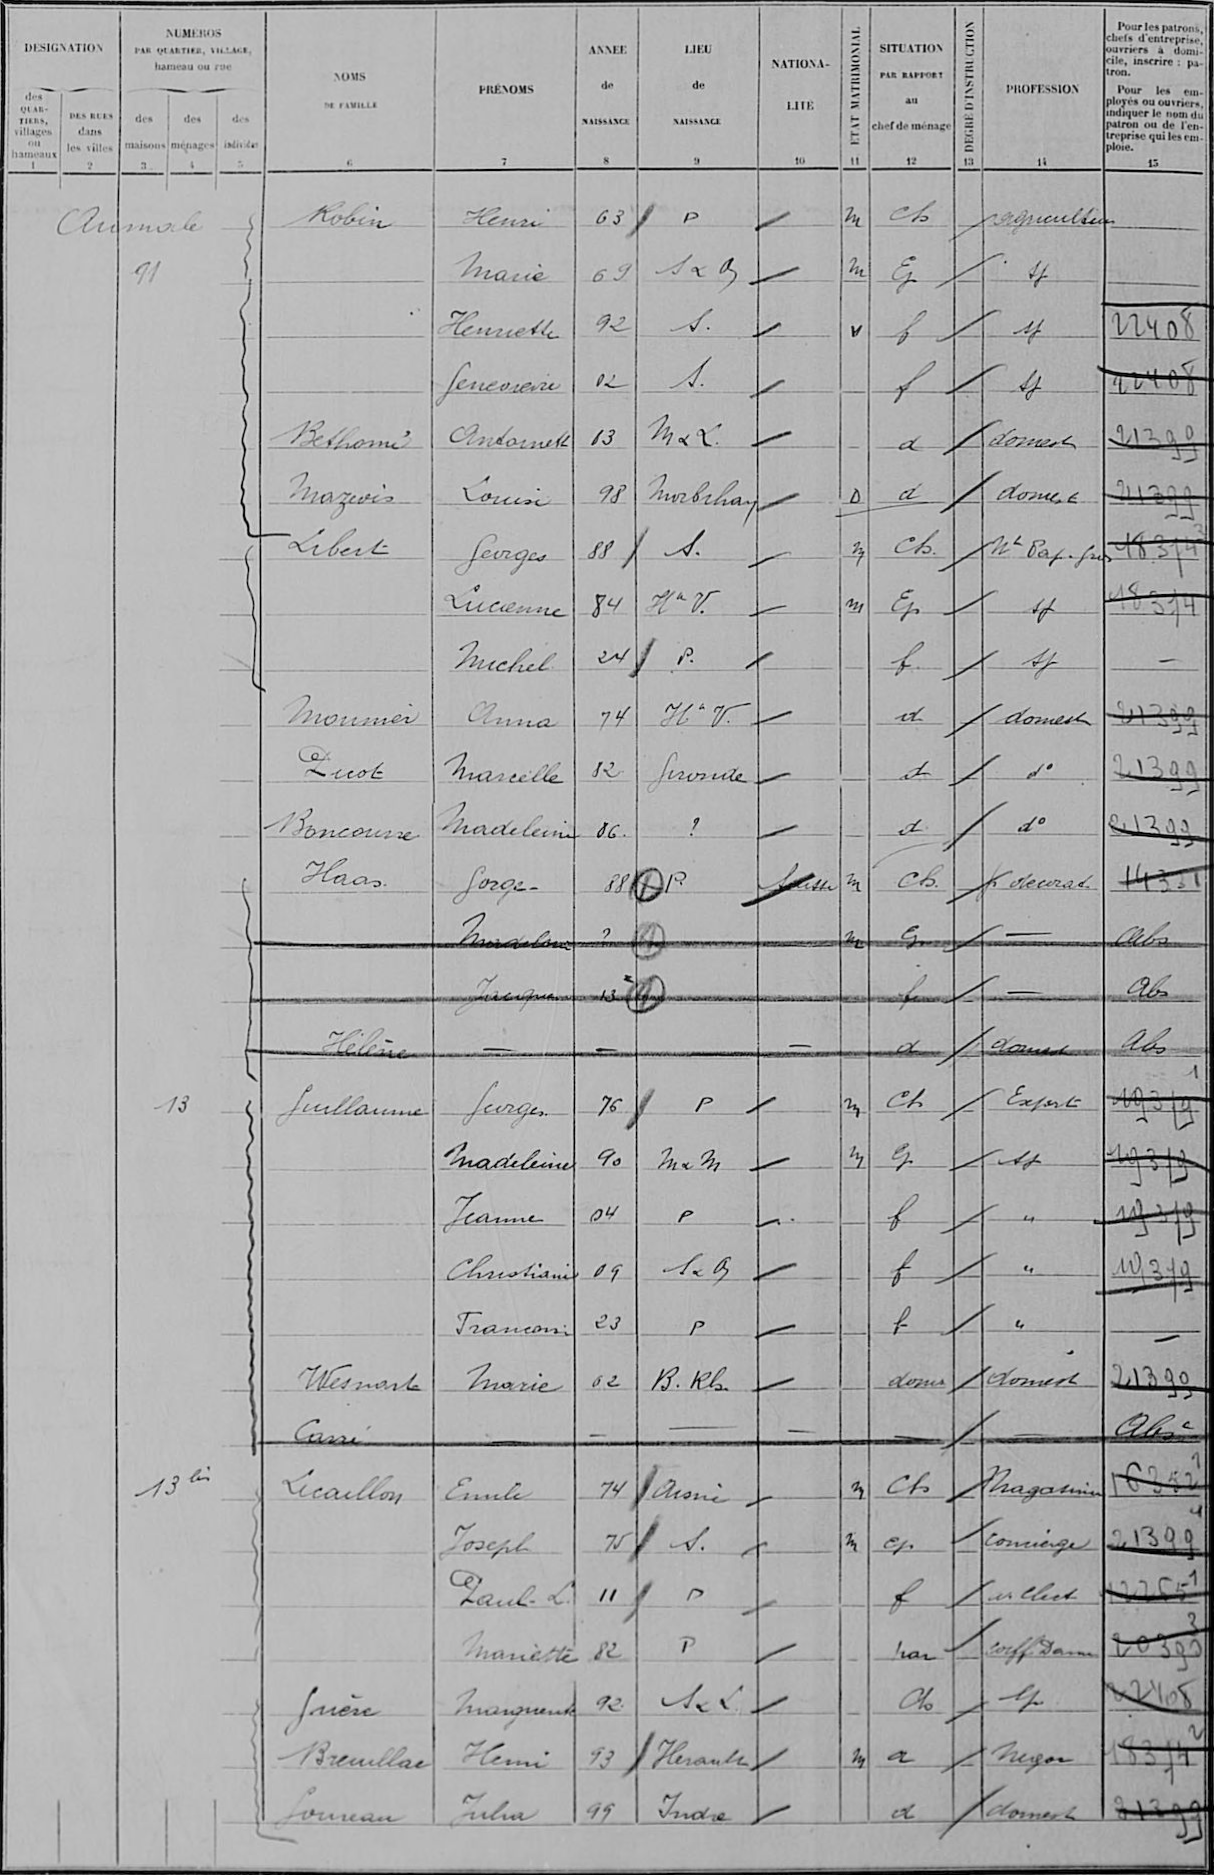Robin/Henri/63/P/¤/M/Ch/¤/agriculteur/¤
¤/Marie/69/S & O/¤/M/Ep/¤/sp/¤
¤/Henriette/92/S /¤/v/f/¤/sp/2208
¤/Geneviève/02/S /¤/¤/f/¤/sp/22408
Bethomé/Antoinette/13/M & L /¤/¤/a/¤/domest/21399
Mazeois/Louise/98/Morbihan/¤/D/d/¤/domest/21399
Libert/Georges/88/S /¤/m/Ch /¤/Nt Pap gris/18374
¤/Lucienne/84/Hte V /¤/m/Ep/¤/sp/18374
¤/Michel/24/P /¤/¤/f /¤/sp/¤
Mounier/Anna/74/Hte V /¤/¤/d/¤/domest/21399
Picot/Marcelle/82/Gironde/¤/¤/d/¤/d°/21399
Boncourre/Madeleine/86/¤/¤/¤/d/¤/d°/21399
Haas/Gorge/88/P/Suisse/M/Ch/¤/p decorat/14331
¤/Madeleine/¤/¤/¤/m/Ep/¤/¤/abs
¤/Jacques/13/¤/¤/¤/f/¤/¤/Abs
Hélène/¤/¤/¤/¤/¤/d/¤/domest/Abs
Guillaume/Georges/76/P/¤/m/Ch/¤/Expert/19379
¤/Madeleine/90/M & M/¤/M/Ep/¤/Sp/19379
¤/Jeanne/04/P/¤/¤/f/¤/"/19379
¤/Christiane/09/S & M/¤/¤/f/¤/"/19379
¤/Françoise/23/P/¤/¤/f/¤/"/¤
Wesmark/Marie/02/B Rh/¤/¤/dom/¤/domest/21399
Carri/¤/¤/¤/¤/¤/¤/¤/¤/Abs
Lecaillon/Emile/74/Aisne/¤/M/Ch/¤/Magasinier/16352
¤/Joseph/75/S /¤/M/ep/¤/concierge/21399
¤/Paul L/11/P/¤/¤/f/¤/a clerc/12265
¤/Mariette/82/P/¤/¤/par/¤/coiff Dames/20390
Imère/Marguerite/92/S & L/¤/¤/Ch/¤/Ep/22408
Breuillac/Henri/93/Herault/¤/M/a/¤/Negoc/18374
Gourreau/Julia/99/Indre/¤/¤/d/¤/domest/21399
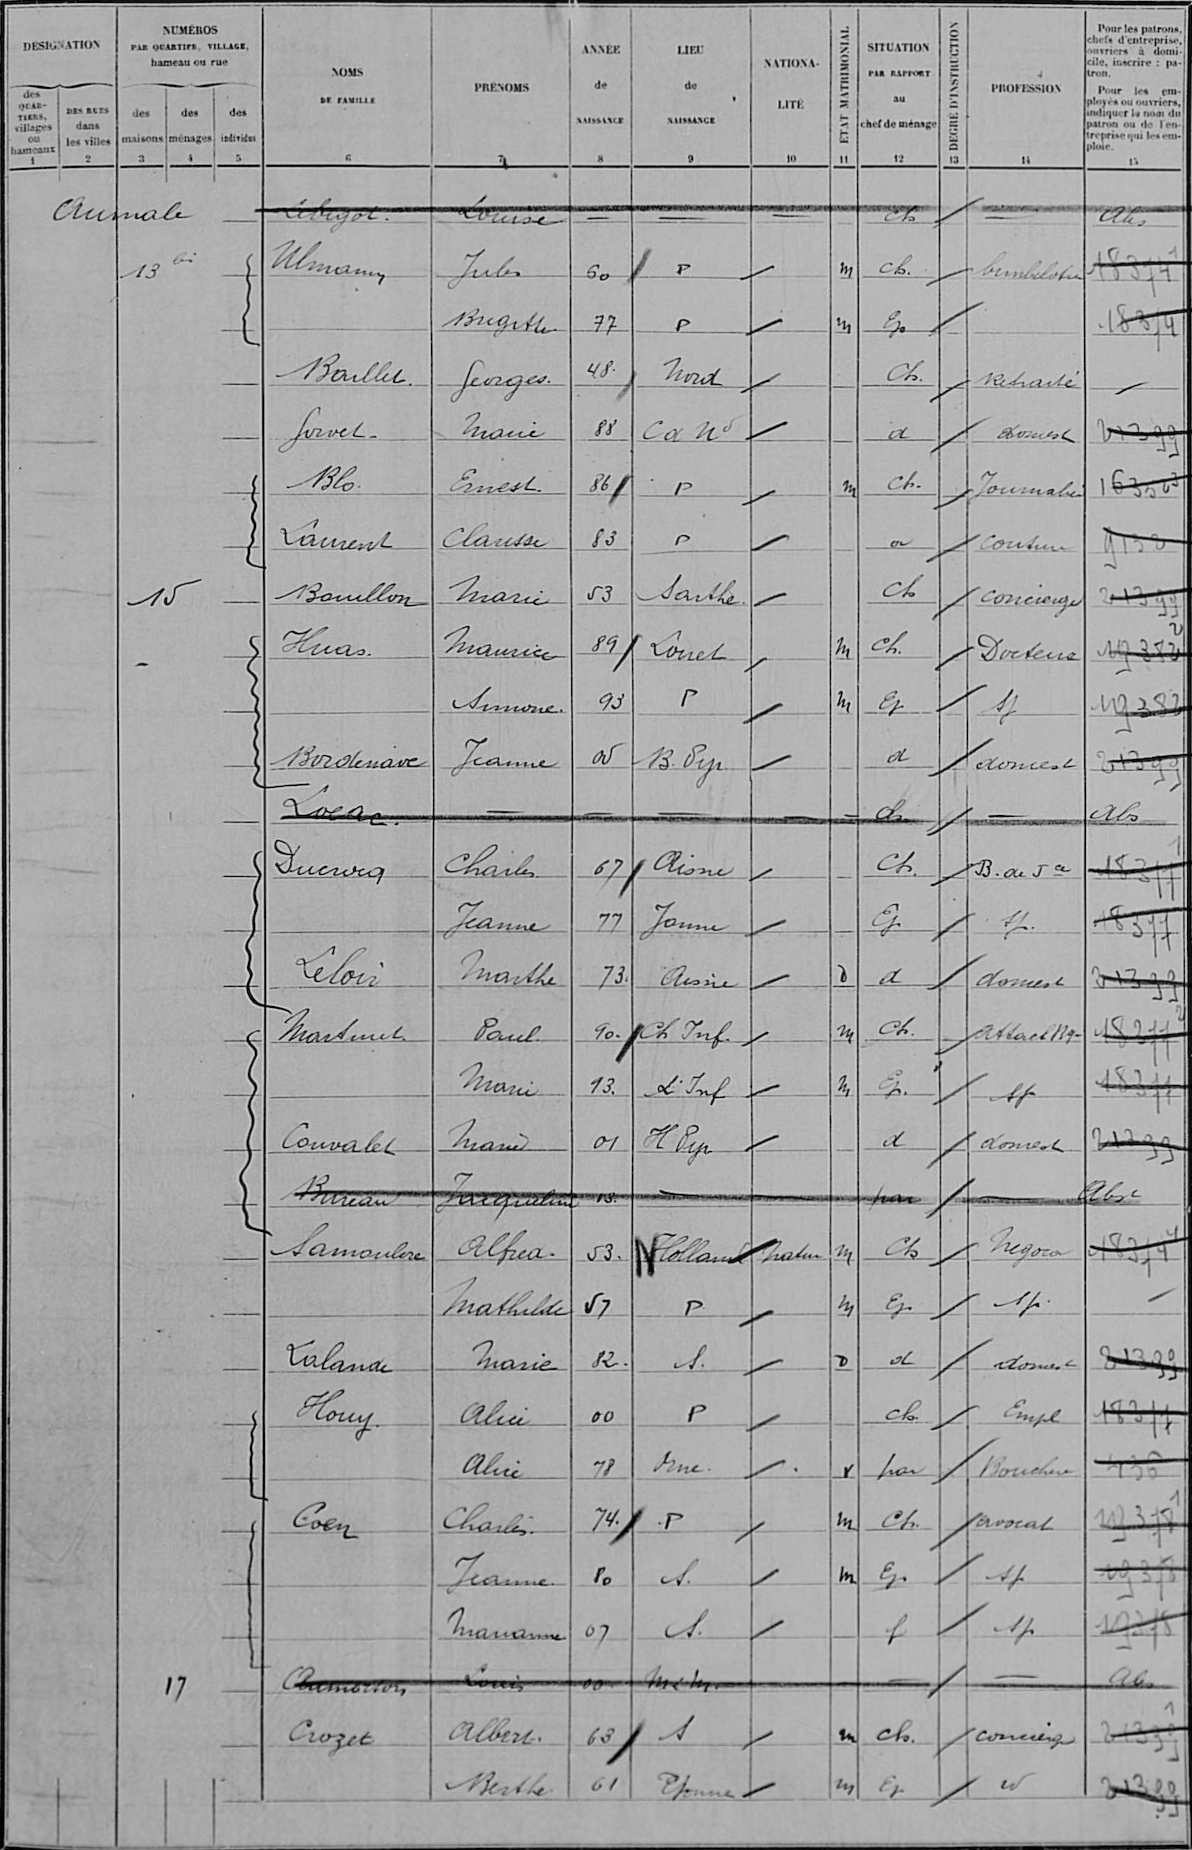Abigot/Louise/¤/¤/¤/¤/Ch/¤/¤/Abs
Ulman/Jules/60/P/¤/M/ch /¤/bimbelotier/18374
¤/Brigitte/77/P/¤/m/Ep/¤/¤/1837
Baillet/Georges/48/Nord/¤/¤/Ch /¤/REtraité/¤
Gorvet/Marie/88/C d Nd/¤/¤/d/¤/domest/21399
Blo/Ernest/86/P/¤/M/Ch /¤/Journalier/16352
Laurent/Clarisse/83/P/¤/¤/a/¤/couture/9132
Bouillon/Marie/53/Sarthe/¤/¤/Ch/¤/concierge/21399
Huas/Maurice/89/Loiret/¤/M/Ch /¤/Docteur/19382
¤/Simone/93/P/¤/M/Ep/¤/Sp/19382
Bordinave/Jeanne/00/B Pyr/¤/¤/d/¤/domest/21399
Loeac/¤/¤/¤/¤/¤/Ch/¤/¤/Abs
Ducrocq/Charles/67/Aisnes/¤/¤/Ch /¤/B de Jce/18377
¤/Jeanne/77/Yonne/¤/¤/Ep/¤/sp/18377
Leloir/Marthe/73/Aisne/¤/o/a/¤/domest/21399
Martinet/Paul/90/Ch Inf /¤/M/Ch/¤/Attach Bq/18377
¤/Marie/13/L Inf/¤/M/Ep /¤/sp/18377
Convalet/Marie/01/H Pyr/¤/¤/d/¤/domest/21399
Bureau/Jacqueline/13/¤/¤/¤/par/¤/¤/Abs
Samoulere/Alfred/53/Hollande/Natur/M/Ch/¤/Negoc/183374
¤/Mathilde/57/P/¤/M/Ep/¤/Sp /¤
Lalande/Marie/82/S /¤/D/d/¤/domest/21399
Houy/Alice/00/P/¤/¤/Ch/¤/Empl/18377
¤/Alice/78/Orne/¤/V/par/¤/Bouchère/436
Coen/Charles/74/P/¤/m/Ch/¤/avocat/19378
¤/Jeanne/80/S /¤/m/Ep/¤/sp/19378
¤/Marianne/07/S /¤/¤/f/¤/Sp/19378
Aumesron/Louis/00/M & M/¤/¤/¤/¤/¤/Abs
Crozet/Albert/63/S/¤/m/Ch/¤/concierge/21399
¤/Berthe/61/Somme/¤/m/Ep/¤/id/21399